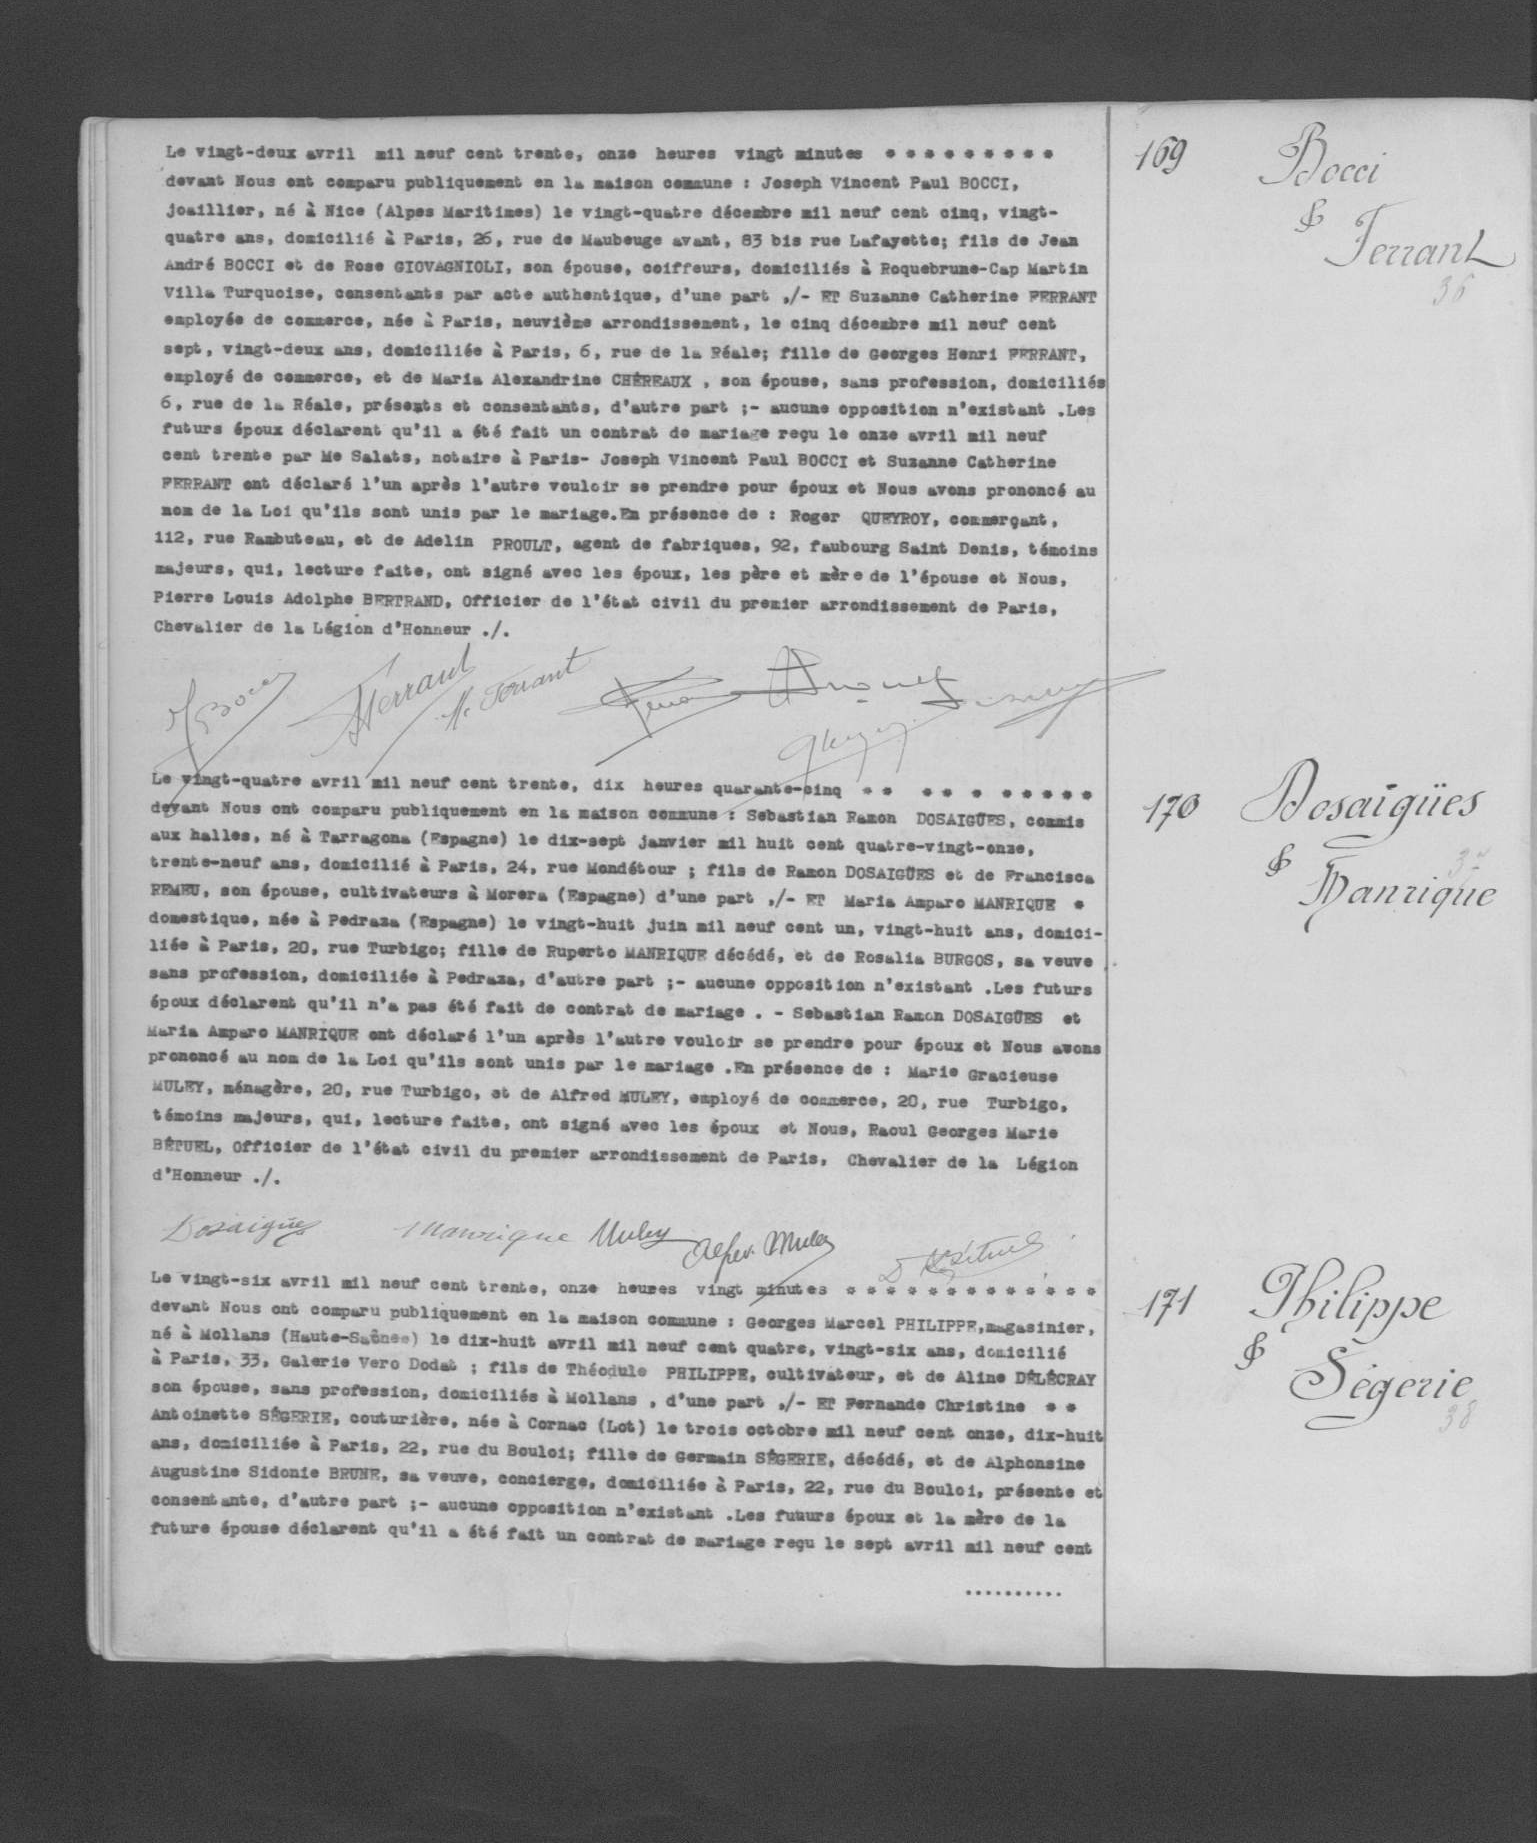Le vingt-deux avril mil neuf cent trente, onze heures cingt minutes ****
devant Nous ont comparu publiquement en la maison commune: Joseph Vincent Paul BOCCI,
joaillier, né à Nice (Alpes Maritimes) le vingt-quatre décembre mil neuf cent cinq, vingt-
quatre ans, domicilié à Paris, 26, rue de Maubeuge avant, 83 bis rue Lafayette; fils de Jean
André BOCCI et de Rose GIOVAGNIOLI, son épouse, coiffeurs, domiciliés à Roquébrune-Cap Martin
Villa Turquoie, consentants par acte authentique, d'une part ,/-ET Suzanne Catherine FERRANT
employée de commerce, née à Paris, neuvième arrondissement, le cinq décembre mil neuf cent
sept, vingt-deux ans, domiciliée à Paris, 6, rue de la Réale; fille de Georges Henri FERRANT,
employé de commerce, et de Maria Alexandrine CHEREAUX, son épouse, sans profession, domiciliés
6, rue de la Réale, présents et consentants, d'autre part ;-aucune oppsoition n'existant .Les
futurs époux déclarent qu'i as été fait un contrat de mariqge reçu le onze avril mil neuf
cent trente par Me Salats, notaire à Paris-Joseph Vincent Paul BOCCI et Suzanne Catherine
FERRANT ont déclaré l'un après l'autre vouloir se prendre pour époux et Nous avons prononcé au
nom de la Loi qu'ils sont unis par le mariage. En présence de: Roger QUEYROY, commerçant,
112, rue Rambuteau, et de Adelin PROULT, agent de fabriques, 92, faubourg Saint Denis, témoins
majeurs, qui, lecture faite, ont signé avec les époux, les père et mère de l'épouse et Nous,
Pierre Louis Adolphe BERTRAND, Officier de l'état civil du premier arrondissement de Paris,
Chevalier de la Légion d'Honneur ./.
Le vingt-quatre avril mil neuf cent trente, dix heures quarante-cinq ****
devant Nous ont comparu publiquement en la maison commune: Sebastien Ramon DOSAIGUES, commis
aux halles, né à Tarragona (Espagne) le dix-sept janvier mil huit cent quatre-vingt-onze,
trente-neuf ans, domicilié à Paris, 24, rue Mondétour; fils de Ramon DOSAIGUES et de Francisca
REMEU, son épouse, cultivateurs à Morera (Espagne) d'une part ,/-ET Maria Apparo MANRIQUE *
domestique, née à Pedraza (Espagne) le vingt-huit juin mil neuf cent un, vingt-huit ans, domici-
liée à Paris, 20, rue Turbigo; fille de Ruperto MANRIQUE décédé, et de Rosalia BURGOS, sa veuve
sans profession, domiciliée à Pedraza, d'autre part ;-aucune opposition n'existant. Les futurs
époux déclarent qu'il n'a pas été fait de contrat de mariage .-Sebastian Ramon DOSAIGUES et
Maria Amparo MANRIQUE ont déclaré l'un après l'autre vouloir se prendre pour époux et Nous avons
prononcé au nom de la Loi qu'ils sont unis par le mariage. En présence de: Marie Gracieuse
MULEY, ménaguère, 20, rue Turbigo, et de Alfred MULEY, employé de commerce, 20, rue Turbigo,
témoins majeurs, qui, lecture faite, ont signé avec les époux et Nous, Raoul Georges Marie
BETUEL, Officier de l'état civil du premier arrondissement de Paris, Chevalier de la Légion
d'Honneur ./.
Le vingt-six avril mil neuf cent trente, onze heures vingt minutes ****
devant Nous ont comparu publiquement en la maison commune: Georges Marcel PHILIPPE, magasinier,
né à Mollans (Haute-Saônee) le dix-huit avril mil neuf cent quatre, vingt-six ans, domicilié
à Paris, 33, Galerie Vero Dodat; fils de Théodule PHILIPPE, cultivateur, et de Aline DELECRAY
son épouse, sans profession, domiciliés à Mollans, d'une part ,/-ET Fernande Christine * *
Antoinette SEGERIE, couturière, née à Cornac (Lot) le trois octobre mil neuf cent onze, dix-huit
ans, domicilié à Paris, 22, rue du Bouloi; fille de Germain SEGERIE, décédé, et de Alphonsine
Augustine Sidonie BRUNE, sa veuve, concierge, domiciliée à Paris, 22, rue du Bouloi, présente et
consentante, d'autre part ;-aucune opposition n'existant. Les futurs époux et la mère de la
future épouse déclarent qu'il a été fait un contrat de mariage reçu le sept avril mil neuf cent
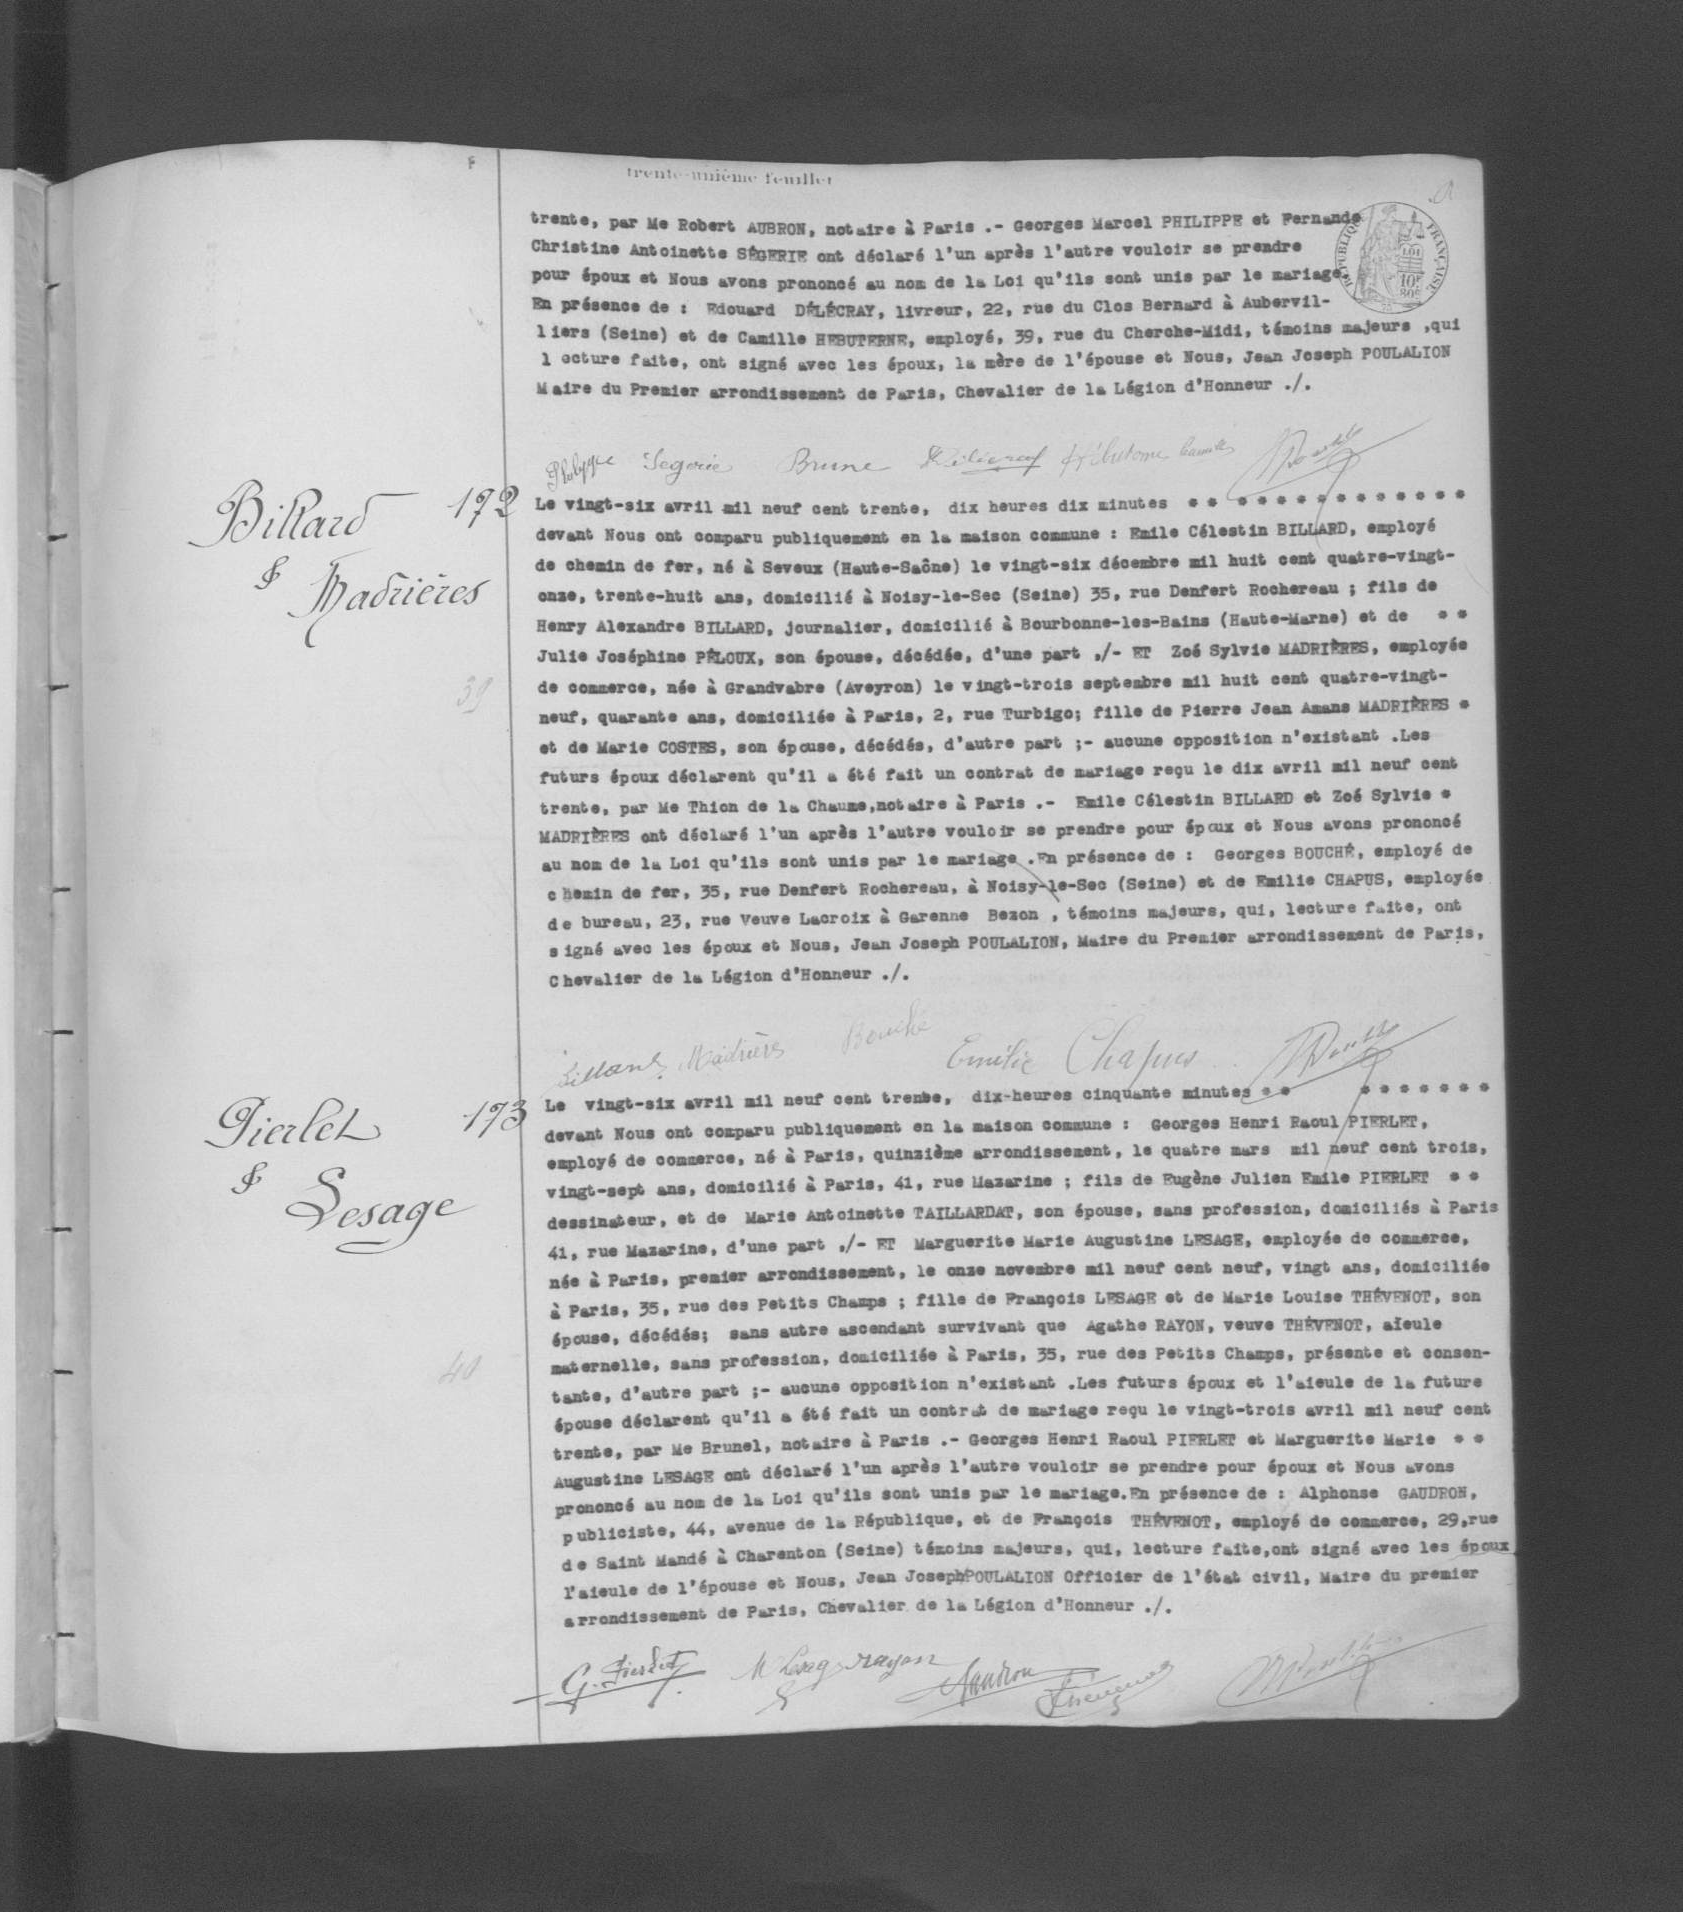trente, par Me Robert AUBRON, notaire à Paris .-Georges Marcel PHILIPPE et Fernance
Christine Antoinette SEGERIE ont déclaré l'un après l'autre vouloir se prendre
pour époux et Nous avons prononcé au nom de la Loi qu'ils sont unis par le mariage.
En présence de: Edouard DELECRAY, livreuer, 22, rue du Clos Bernard à Aubervil-
liers (Seine) et de Camille HEBUTERNE, employé, 39, rue du Cherche-Midi, témoins majeur ,qui
lecture faite, ont signé avec les époux, la mère de l'épouse et Nous, Jean Joseph POULALION
Maire du Premier arrondissement de Paris, Chevalier de la Légion d'Honneur ./.
Le vingt-six avril mil neuf cent trente, dix heures dix minutes ****
devant Nous ont comparu publiquement en la maison commune: Emile Célestin BILLARD, employé
de chemin de fer, né à Seveux (Haute-Saône) le vingt-six décembre mil huit cent quatre-vingt-
onze, trente-huit ans, domicilié à Noisy-le-Sec (Seine) 35, rue Denfert Rochereau; fils de
Henry Alexandre BILLARD, journalier, domicilié à Bourbonne-les-Bains (Haute-Marne) et de * *
Julie Joséphine PELOUX, son épouse, décédée, d'une part ,/-ET Zoé Sylvie MADRIERES, employée
de commerce, née à Grandvabre (Aveyron) le vingt-trois septembre mil huit cent quatre-vingt-
neuf, quarante ans, domiciliée à Paris, 2, rue Turbigo; fille de Pierre Jean Amans MADRIERES *
et de Marie COSTES, son épouse, décédés, d'autre part ;-aucune opposition n'existant. Les
futurs époux déclarent qu'il a été fait un contrat de mariage reçu le dix avril mil neuf cent
trente, par Me Thion de la Chaume, notaire à Paris .-Emile Célestin BILLARD et Zoé Sylvie *
MADRIERES ont déclaré l'un après l'autre vouloir se prendre pour époux et Nous avons prononcé
au nom de la Loi qu'ils sont unis par le mariage. En présence de: Georges BOUCHE, empoyé de
chemin de fer, 35, rue Denfert Rochereau, à Noisy-le-Sec (Seine) et de Emilie CHAPUS, employée
de bureau, 23, rue Veuve Lacroix à Garenne Bezon, témoins majeurs, qui, lecture faite, ont
signé avec les époux et Nous, Jean Joseph POULALION, Maire du Premier arrondissement de Paris,
Chevalier de la Légion d'Honneur ./.
Le vingt-six avril mil neuf cent trente, dix-heures cinquante minutes ****
devant Nous ont comparu publiquement en la maison commune: Georges Henri Raould PIERLET,
employé de commerce, né à Paris, quizième arrondissement, le quatre mars mil neuf cent trois,
vingt-sept ans, domicilié à Paris, 41, rue Mazarine: fils de Eugène Julien Emile PIERLET * *
dessinateur, et de Marie Antoinette TAILLARDAT, son épouse, sans profession, domiciliés à Paris
41, rue Mazarine, d'une part ,/-Et Marguerite Marie Augustine LESAGE, empoyée de commerce,
née à Paris, premier arrondissement, le onze novembre mil neuf cent neuf, vingt ans, domiciliée
à Paris, 35, rue des Petits Champs; fille de François LESAGE et de Marie Louise THEVENOT, son
épouse, décédés; sans autre ascendant survivant que Agathe RAYON, veuve THEVENOT, aïeule
maternelle, sans profession, domiciliée à Paris, 35, rue des Petits Champs, présente et consen-
tante, d'autre part ;-aucune opposition n'existant. Les futurs époux et l'aieule de la future
épouse déclarent qu'il a été fait un contrat de mariage reçu le vingt-trois avril mil neuf cent
trente, par Me Brunel, notaire à Paris .-Georges Henri Raoul PIERLET et Marguerite Marie * *
Augustine LESAGE ont déclaré l'un après l'autre vouloir se prendre pour époux et Nous avons
prononcé au nom de la Loi qu'ils sont unis par le mariage. En présence de: Alphonse GAUDRON,
publiciste, 44, avenu de la République, et de François THEVENOT, employé de commerce, 29, rue
de Saint Mandé à Charenton (Seine) témoins majeurs, qui, lecture faite, ont signé avec les époux
l'aieule de l'épouse et Nous, Jean Joseph POULALION Officier de l'état civil, Maire du premier
arrondissement de Paris, Chevalier de la Légion d'Honneur ./.